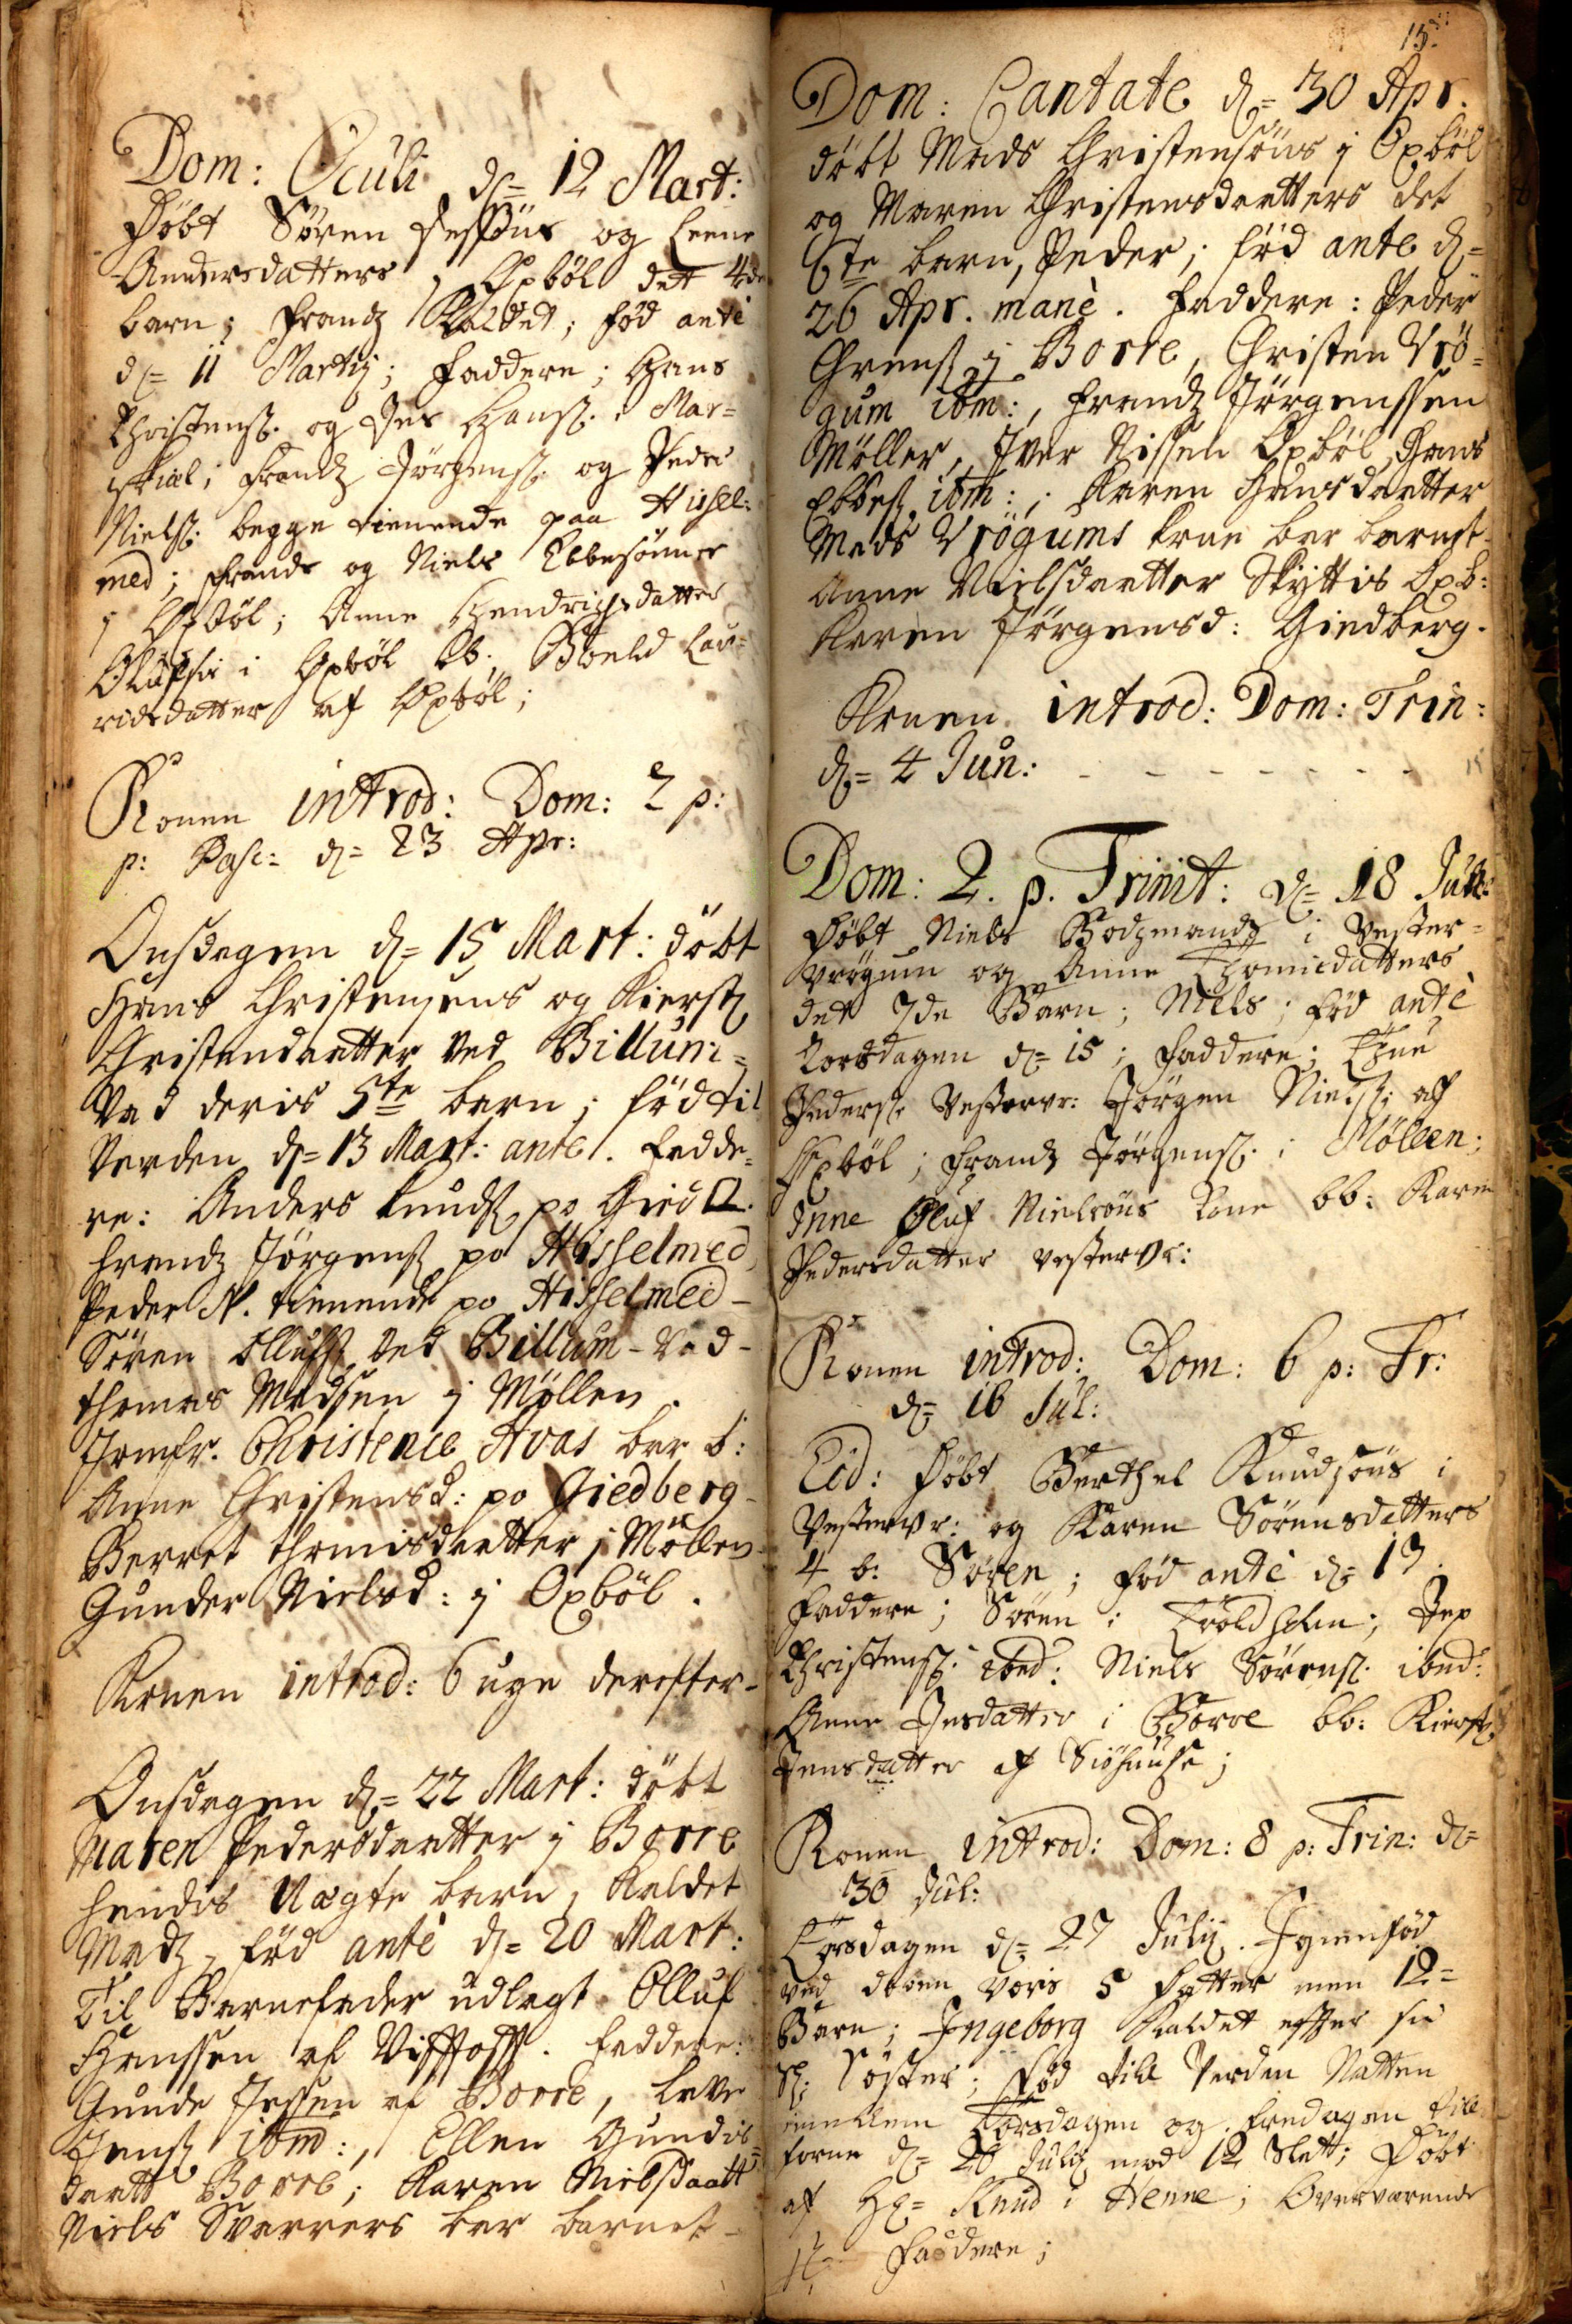Dom: Oculi d= 12 Mart:
Døbt Søren Jessøns og Leene
Andersdatters i Oxbøl det 4de
barn; Frandz Kaldet; fød ante
d= 11 Martij; Faddere; Hans
Christens og Jes Hans i Mar¬
skiæl; Frandz Jørgensen og Peder
Niels begge tienende paa Hissel¬
med; Frands og Niels Ebbesøn##
j Oxbøl; Anne Hendichsdatter
Oluffs i Oxbøl ##; Boeld Lav¬
ridsdatter af Oxbøl;
Konen introd: Dom: 2 p:
p: Pasc: d= 23 Apr:
Onsdagen d= 15 Mart:  døbt
Hans Christensens og Kierst
Christensdatter ved Billum¬
Vad deris 5te barn; fød til
Verden d=13 Mart: ante. Fadde¬
re: Anders Knuds po Gied ##.
Frandz Jærgens po Hisselmed
Peder N. tienende po Hisselmed -
Søren Olufs ved Billum-Vad -
Thomas Madsen j Møllen,
Jomfr. Christence Hvas bar b.
Anne Christensd. po Giedberg,
Berret Thomisdatter i Møllen.
Gunder Nielsd: i Oxbøl.
Konen introd: 6 uge derefter.
Onsdagen d= 22 Mart: døbt
Maren Pedersdaatter j Borre
hendis Uægte barn, kaldet
Madz, fød ante d= 20 Mart:
Til Barnefader udlagt Olluf
Hanssøn af Vifftoft. Faddere:
Gunde Jessen af Borre, Lave
Jensen ibm: , Ellen Gundis
daatter Borre; Karen Nielsdaatt
Niels Svarrers bar barnet:
15
15
Dom: Cantate d= 30 Apr.
døbt Mads Christensøns j Oxbøl
og Maren Christensdaatters det
6te barn, Peder; fød ante d=
26 Apr. manè. Faddere: Peder
Chrens j Borre, Christen Vrø¬
gum ibm:; Frandz Jørgenssen
Møller, Iver Nissen Oxbøl Hans
Ebbes ibm:; Karen Hansdaatter
Mads Vrøgums Kone bar barnet.
Anne Nielsdaatter Skyttes Oxb:
Karen Jørgensd: Giedberg.
Konen introd: Dom: Trin:
d= 4 Jun:
Dom: 2. p. Trinit: d=18 Ju##:
Døbt Niels Bodzmands i Vester
Vrøgum og Anne Thomisdatters
det 7de Barn; Niels; fød ante
Torsdagen d= 15; Faddere; Thue
Peders Vestervr: Jørgen Niels af
Oxbøl; Frandz Jørgens i Møllen:
Anne Oluf Nielsøns Kone bb: Karen
Pedersdatter Vestervr:
Konen introd: Dom: 6 p: Tr:
d=16 Jul:
Eod. Døbt Berthel Knudsøns i
Vestervr: og Karen Sørensdatters
4 b: Søren; fød ante d= 17.
Faddere;  Søren i Troldholm; Jep
Christens ibm: Niels Søren ibm:
Anne Jesdatter i Borrre bb: Kierst
Jensdatter af Siøhuuse;
Konen introd: Dom: 8 p: Trin: d=
30 Jul:
Torsdagen d= 27 Julij Igienfød
ved Doben voris 5 Datter min 12=
Barn; Ingeborg kaldet effter sin
Sl. Søster; fød til Verden Natten
imellem Torsdagen og Fredagen til
forne d= 20 JuliJ mod 12 Slet; Døbt
af Hr= Knud i Henne; Overværende
s Faddere;